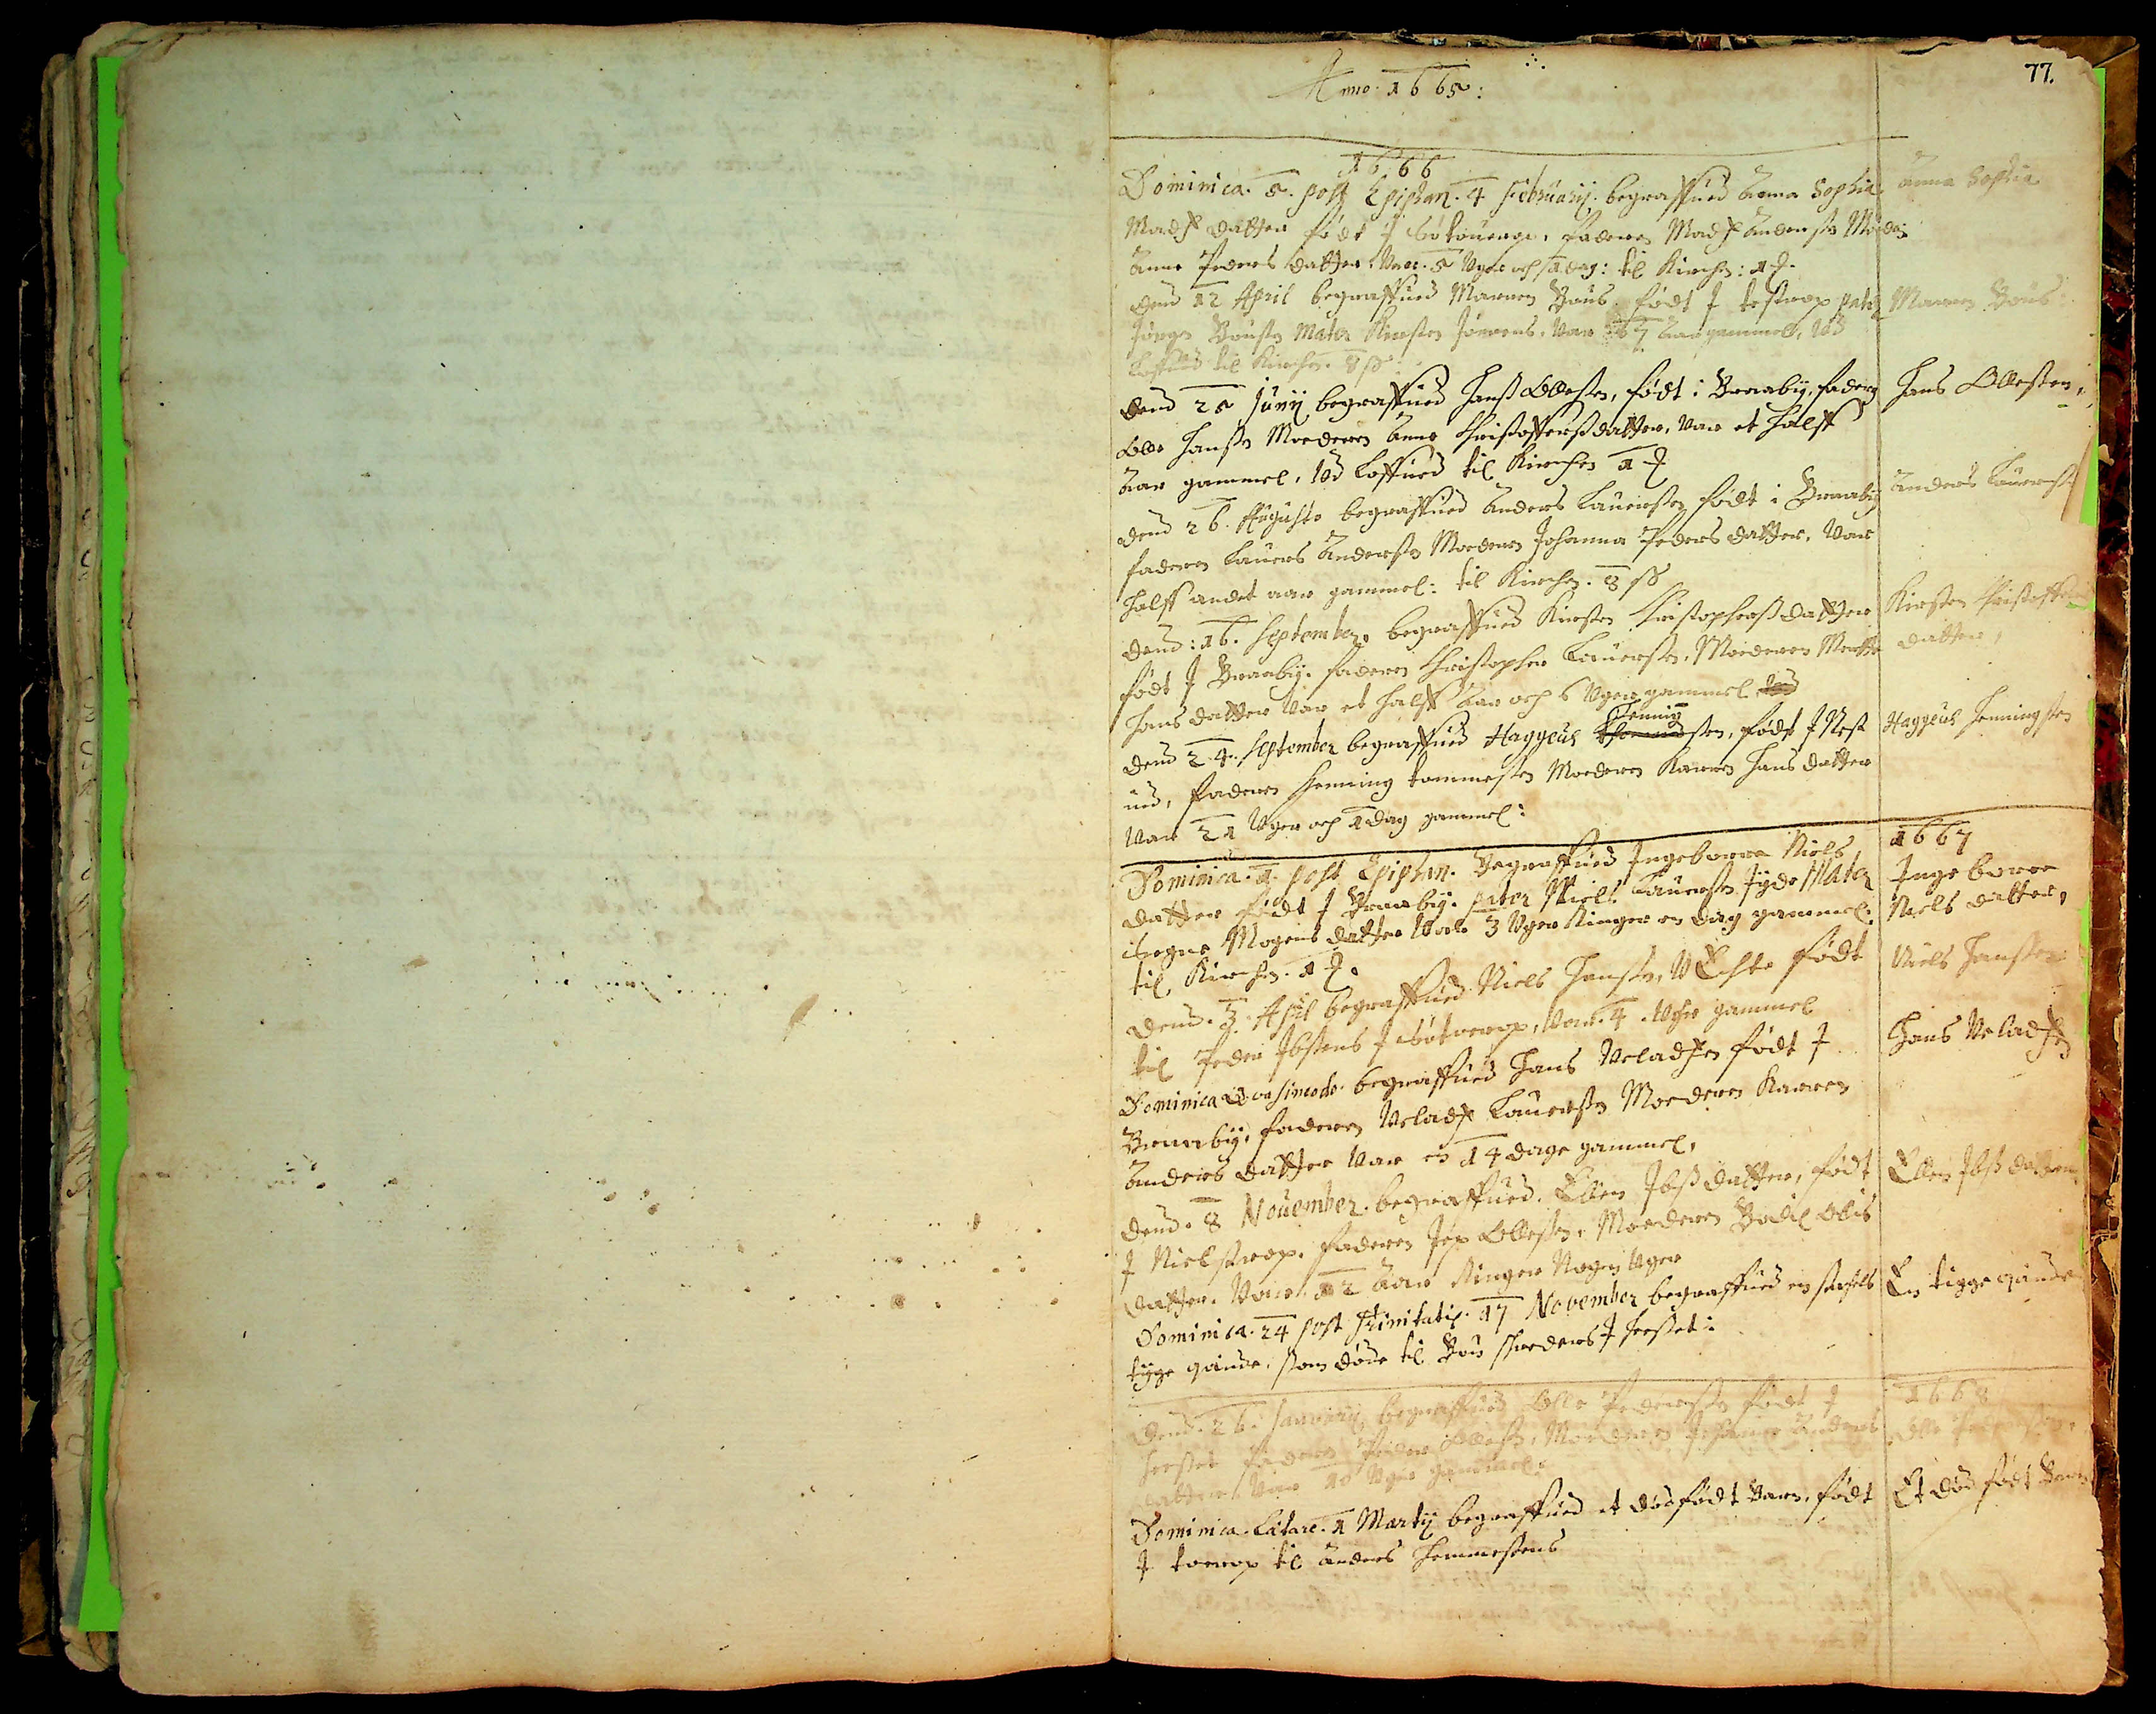Anno 1665
1666
Dominica 5. post Epiphan. 4. February begraffued Anna Sophia
Mads Datter født J Søtorup, Faderen Mads Anders Moed##
Anna Peders Datter. Var 5 Uger och 1 Dag: til Kirchen: 1Mk.
77.
Anna Sophia
Dend. 12 April begraffued Marren Bous. født J Testrop Pater
Jørgen Bousen Mater Kirsten Jørrens. Var ## 7 Aar gammel, ##
loffued til Kirchen 8 sk:
Marren Bous.
Dend. 25 Juny begraffued Hans Boesen, født i Braaby, Faderen
Boe Hansen Moederen Anne Christoffersdatter, var et halff
Aar gammel. Ud loffued til Kirchen 1Mk.
Hans Boessen
Dend 26 Augusti begraffued Anders Lauersen født i Braaby,
Faderen Lauers Andersøn Moederen Johanna Pedersdatter. Var
halff andet Aar gammel: til Kirchen. 8 sk.
Anders Lauersen
Dend: 16. September begraffued Kirsten Christophersdatter
født J Braaby. Faderen Christopher Lauersen. Moederen Mette
Hansdatter. Var et halft Aar och 6 Uger gammel. ##
Kirsten Christoffers
datter
Henning
Dend 24. September begraffued Haggeus## ##søn, født J Nest
ved. Faderen Henning Tommesen Moederen Karen Hansdatter.
Vare 21 Uger och 1 Dag gammel:
Haggeus## Henningsen
Dominica. 1. post Epiphan. begraffued Ingeborre Niels
datter født J Braaby. Pater Niels Lauersøn Jyde Mater
Seigne Mogensdatter. Vare 3 Uger ringer en Dag gammel,
til Kirchen 1Mk.
1667
Ingeborre
Nielsdatter,
Dend. 3. April begraffued Niels Hansøn Uechte, født
til Peder Jbsens J Søtoerup. Vare. 4. Uger gammel
Niels Hansen
Dominica Quasimodo. begraffued Hans Veladsen, født J
Braaby, Faderen Velads Lauerssen, Moederen Karren
Andersdatter. Var en 14 Dage gammel.
Hans Veladsøn
Dend. 8 Nouember. begraffued Ellen Jbsdatter, født
J Nielstrop, Faderen Jep Ollesen, Moederen Bodil Ollis
datter. Var ## 2 Aare Ringer Nogen Uger.
Ellen Jbsdatter
Dominica. 24. post Trinitatis. 17 November begraffued en Stachels
tigge Quinde, som døde til Bou Skreders## J Hirset:
En Tigge Quinde
Dend. 26. January begraffued Olle Pedersen født J
Hirset, Faderen Peder Ollesøn, Moederen Johanne Anders
datter. Var 20 Uger gammel:
1668 
Olle Pedersøn
Dominica Lætare. 1.Marty, begraffued et dødfødt Barn, født
J Torup til Anders Hammersens##
Et død født barn
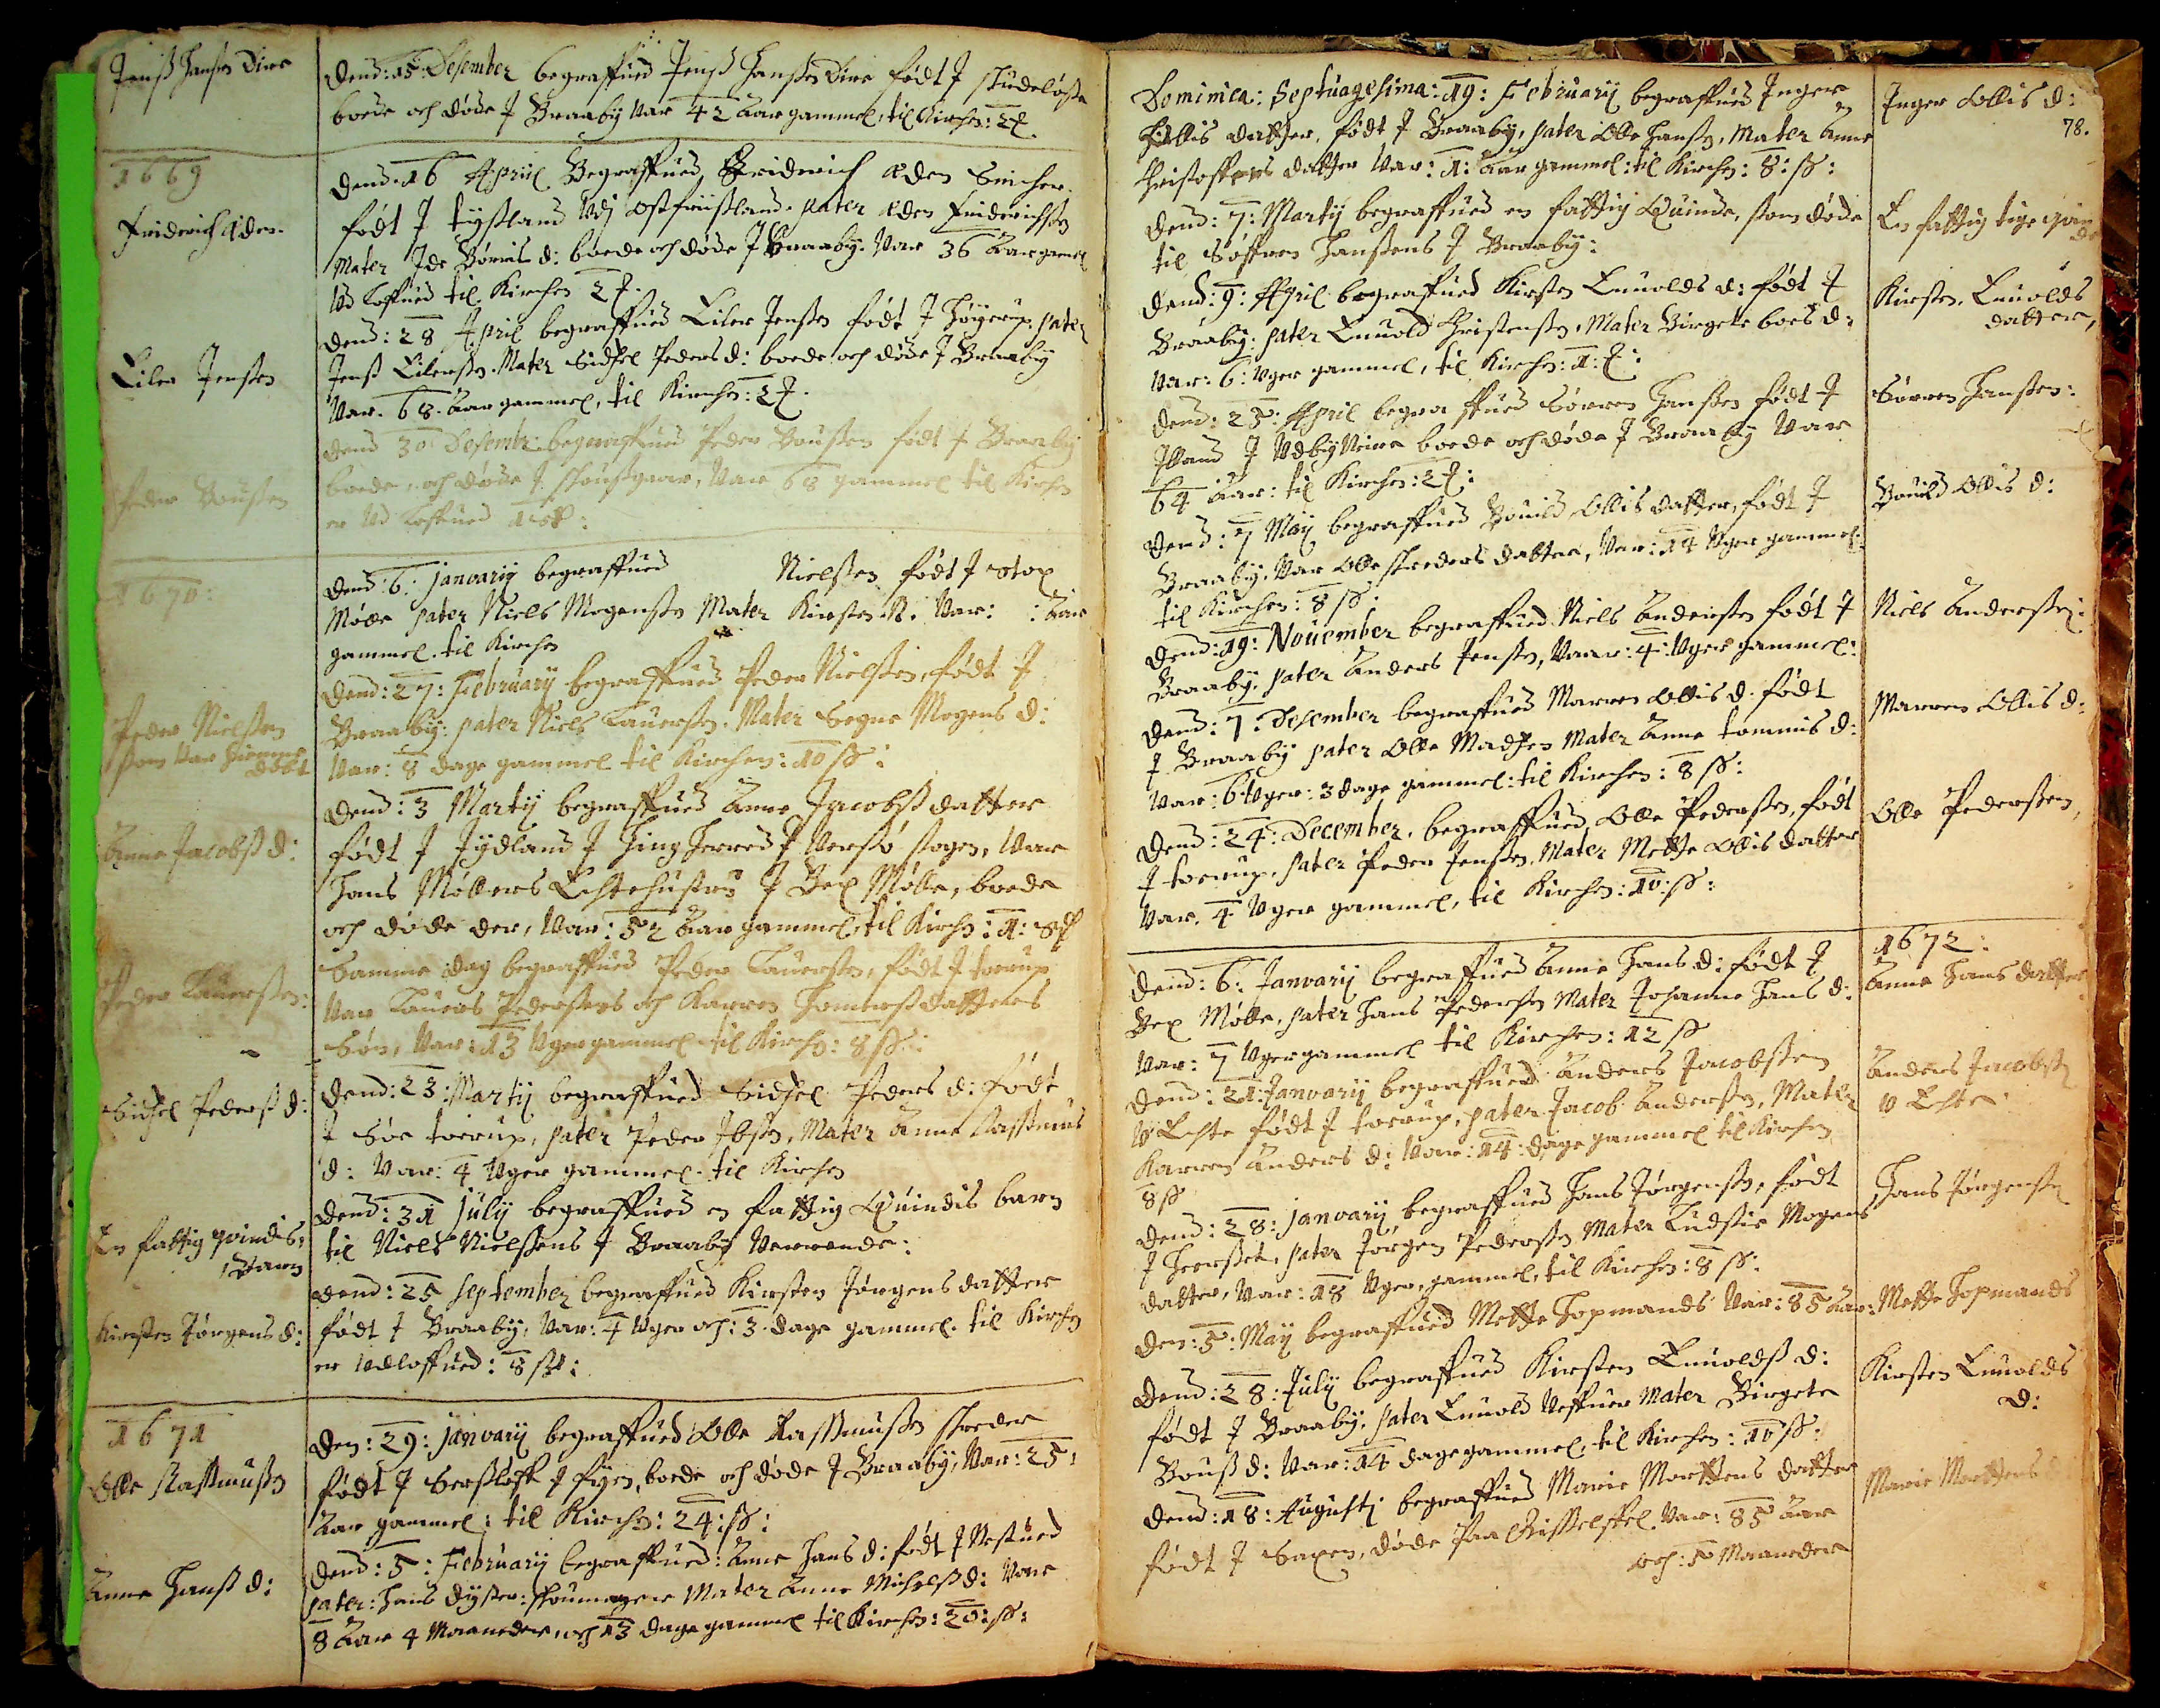Jens Hansøn Dire##
Dend: 15 December begraffued Jens Hansen Dire## født J Skudeløse
boede och døde J Braaby. Var 42 Aar gammel, til Kirchen: 2 Mk.
1669 
Friderich Olles##
Dend. 16. Apriil Begraffued Friderich Olles## Snicher
født J Tyskland Udi Ostfriisland. Pater Olles## Friderichsen,
Mater Ide Bømisd: boede och døde J Braaby. Vare 36 Aar gammel.
Ud Loffued til Kirchen 2 Mk.
Eiler Jensøn 
Dend: 28 April begraffued Eiler Jensøn, født J Høijerup. Pater
Jens Eilersen, Mater Sidsel Pedersd: boede och døde J Braaby.
Var 68 Aar gammel, til Kirche: 2 Mk.
Peder Bousen
Dend 30. December begraffued Peder Bousen født J Braaby,
boede och døde i Skousgaard. Var 68 gammel, til Kirch
en Ud loffued 1Mk. 5 Sk:
1670:
Dend: 6: january begraffued
Nielsøn født J Stox
mølle, Pater Niels Mogensen, Mater Kirsten N. Var:  :Aar
gammel, til Kirchen
Peder Nielsen
som Var hiemme
døbt
Dend: 27: February begraffued Peder Nielsøn, født J
Braaby: Pater Niels Lauerssen, Mater Segne Mogens d.
Var: 8 Dage gammel, til Kirchen: 10 Sk:
Anne Jacobsd:
Dend: 3. Marty begraffued Anna Jacobsdatter
født i Jydland J Hing Herred J Vorsø sogn.## Var
Hans Møllers Echtehustrue J Bex Mølle, boede
och døde der. Var 52 Aar gammel, til Kirchen: 1: ##
Peder Lauersen
Samme dag begraffued Peder Lauersøn, født J Toerup
Var Lauers Pedersøns och Karren Hammersdatters
Søn. Var 13 Uger gammel, til Kirchen: 8 Sk:
Sidsel Pedersd:
Dend: 23: Marty begraffued Sidsel Pedersd: født
J Søe Toerup. Pater Peder Jbsøn, Mater Anna Rassmus
d: Var 4 Uger gammel, til Kirchen
En Fattig Quindis
Barn
Dend: 31 July begraffued en fattig Quindis Barn
til Niels Nielsens J Braaby værende:
Kirsten Jørgensd:
Dend: 25 September begraffued Kirsten Jørgensdatter
født J Braaby. Var: 4 Uger och: 3 Dage gammel. til Kirchen
er Udloffued: 8 Sk:
1671
Olle Rasmussen
Den: 29: January begraffued Olle Rasmussen Skreder,
født J Sersløff J Fyn, boede och døde J Braaby. Var: 25
Aar gammel: til Kirchen: 24: Sk:
Anna Hansd:
Dend: 5: February begraffued Anna Hansd: født i Nestued,
Pater: Hans Dysted:## Skoemager, Mater Anna Michelsd: Var 
 8 Aar 4 Maaneder och 13 Dage gammel, til Kirchen: 20: Sk:
Dominica: Septuagesima: 19: February begraffued Inger
Ollis datter født J Braaby, Pater Olle Hansen, Mater Anna
Christoffersdatter. Var: 1: Aar gammel: til Kirchen: 8: Sk:
78.
Inger Ollisd.
Dend: 7: Marty begraffued en fattig Quinde, som døde
til Søffren Hansens J Braaby:
En fattig tige Quinde
Dend: 9: April begraffued Kirsten Enuoldsd: født J
Braaby: Pater Enuold Christensøn, Mater Birgitte Boesd:
Var: 6: Uger gammel, til Kirchen: 1:Mk:
Kirsten Enuolds
datter,
Dend: 23: April begraffued Sørren Hansøn født J
Jland## J Udby## boede och døde J Braaby. Var
64 Aar: til Kirche: 2 Mk:
Sørren Hansøn
Dend: 7. Maij: begraffued Bouild Ollisdatter, født J
Braaby. Var Olle Skreders Datter. Var: 14 Uger gammel:
Til Kirchen: 8 Sk:
Bouild Ollisd:
Dend: 19: Nouember begraffued Niels Andersøn født J
Braaby: Pater Anders Jensen. Vare: 4: Uger gammelt:
Niels Andersen
Dend: 7: December begraffued Marren Ollisd. født
J Braaby, Pater Olle Madsøn, Mater Anna Tommisd:
Var 6 Uger: 3 Dage gammel: til Kirchen: 8 Sk:
Marren Ollisd:
Dend: 24: December. begraffued, Olle Pederssen, født
J Toerup. Pater Peder Jensen, Mater Mette Ollisdatter,
Var 4 Uger gammel, til Kirchen: 10: Sk:
Olle Pedersen
Dend: 6: January begraffued Anna Hansdatter, født J
Bex Mølle, Pater Hans Pedersen, Mater Johanne Hansd:
Var: 7 Uger gammel, til Kirchen 12 Sk.
1672
Anna Hansdatter
Dend: 21: January begraffued Anders Jacobsen
Uechte født J Toerup, Pater Jacob Andersen, Mater
Karren Andersd: Vare 14: Dage Gammel, til Kirchen:
8 Sk.
Anders Jacobsøn 
uechte
Dend: 28: January begraffued Hans Jørgensen, født
J Heersted,## Pater Jørgen Pedersen , Mater Ludsie Mogens
datter. Var: 18 Uger gammel, til Kirchen: 8 Sk:
Hans Jørgensen
Dend: 5: May begraffued Mette Hopmands Var: 85 Aar:
Mette Hopmands
Dend: 28: July begraffued Kirsten Enuoldsd:
født J Braaby: Pater Enuold Veffuers, Mater Birgete
Bousd: Var: 14 Dage gammel, til Kirchen: 11 Sk:
Kirsten Envolds
d:
Dend: 28: Augusti begraffued Marie Morttens Datter,
født J Saxen##, døde Paa Gisselfel. Var: 85 Aar
och: 5 Maaneder.
Marie Morttensd: